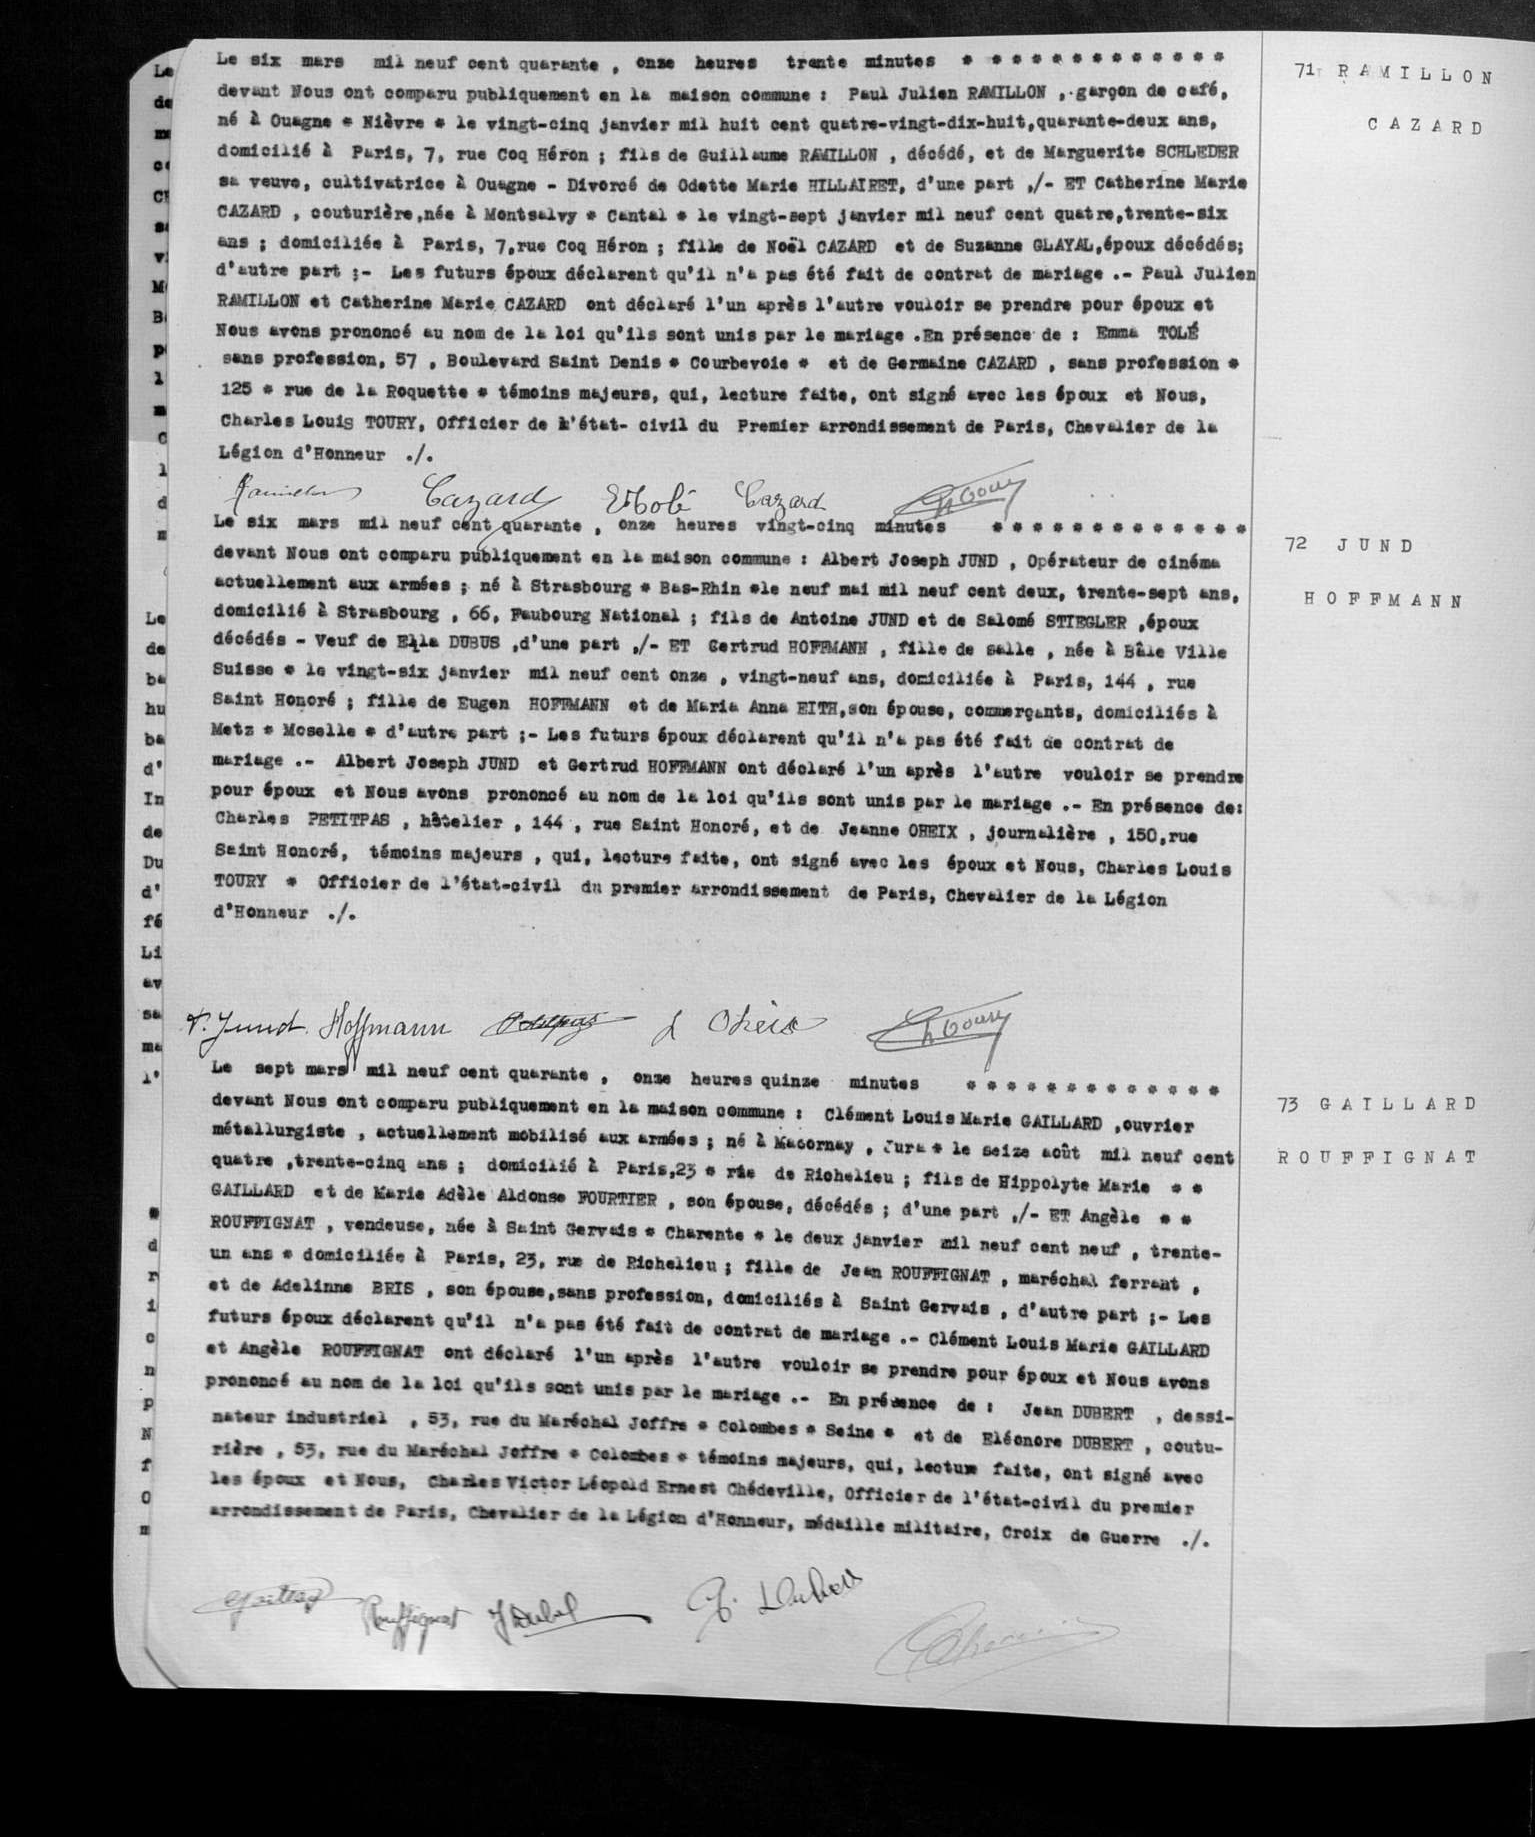Le six mars mil neuf cent quarante, onze heures trente minutes ****
devant Nous ont comparu publiquement en la maison commune: Paul Julien RAMILLON, garçon de café,
né à Ouagne * Nièvre * le vingt-cinq janvier mil huit cent quatre-vingt-dix-huit, quarante-deux ans,
domicilié à Paris, 7, rue Coq Héron; fils de Guillaume RAMILLON, décédé, et de Marguerite SCHLEDER
sa veuve, cultivatrice Ouagne-Divorcé de Odette Marie HILLAIRET, d'une part ,/-Et Catherine Marie
CAZARD, couturière, née à Montsalvy * Cantal * le vingt-sept janvier mil neuf cent quatre, trente-six
ans; domiciliée à Paris, 7, rue Coq Héron; fille de Noël CAZARD et de Suzanne GLAYAL, époux décédés;
d'autre part ;-Les futurs époux déclarent qu'il n'a pas été fait de contrat de mariage .-Paul Julien
RAMILLON et Catherine Marie CAZARD ont déclaré l'un après l'autre vouloir e prendre pour époux et
Nous avons prononcé au nom de la loi qu'ils sont unis par le mariage. En présence de: Emma TOLE
sans profession, 57, Boulevard Saint Denis * Courbevoie * et de Germaine CAZARD, sans profession *
125 * rue de la Roquette * témoins majeurs, qui, lecture faite, ont signé avec les époux et Nous,
Charles Louis TOURY, Officier de l'état-civil du Premier arrondissement de Paris, Chevalier de la
Légion d'Honneur ./.
Le six mars mil neuf cent quarante, onze heures vingt-cinq minutes ****
devant Nous ont comparu publiquement en la maison commune: Albert Joseph JUND, Opérateur de cinéma
actuellement aux armées; né à Strasbourg * Bas-Rhin * le neuf mai mil neuf cent deux, trente-sept ans,
domicilié à Strasbourg, 66, Faubourg National; fils de Antoine JUND et de Salomé STIELGLER, époux
décédés-Veuf de Ella DUBUS, d'une part ,/-ET Gertrud HOFFMAN, fille de salle, née à Bâle Ville
Suisse * le vingt-six janvier mil neuf cent onze, vingt-neuf ans, domiciliée à Paris, 144, rue
Saint Honoré; fille de Eugen HOFFMAN et de Maria Anna EITH, son épouse, commerçants, domiciliés à
Metz * Moselle * d'autre part ;-Les futurs époux déclarent qu'il n'a pas été fait de contrat de
mariage .-Albert Joseph JUND et Gertrud HOFFMAN ont déclaré l'un après l'autre vouloir se prendre
pour époux et Nous avons prononcé au nom de la loi qu'ils sont unis par le mariage .-En présence de:
Charles PETITPAS, hôtelier, 144, rue saint Honoré, et de Jeanne OHEIX, journalière, 150, rue
Saint Honoré, témoins majeurs, qui, lecturs faites, ont signé avec les époux et Nous, Charles Louis
TOURY * Officier de l'état-civil du premier arrondissement de Paris, Chevalier de la Légion
d'Honneur ./.
Le sept mars mil neuf cent quarante, onze heures quinze minutes ****
devant Nous ont comparu publiquement en la maison commune: Clément Louis Marie GAILLARD, ouvrier
métallurgiste, actuellement mobilisé aux armées; né à Macornay, Jura * le seize août mil neuf cent
quatre, trente-cinq ans; domicilié à Paris, 23 * ras de Richelieu; fils de Hippolyte Marie **
GAILLARD et de Marie Adèle Aldonse FOURTIER, son épouse, décédés; d'une part ,/-ET Angèle **
ROUFFIGNAT, vendeuse, née à Saint Gervais * Charente * le deux janvier mil neuf cent neuf, trente-
un ans * domiciliée à Paris, 23, rue de Richelieu; fille de Jean ROUFFIGNAT, maréchal ferrant,
et de Adelinne BRIS, son épouse, sans profession, domiciliés à Saint Gervais, d'autre part:-Les
futurs époux déclarent qu'il n'a pas été fait de contrat de mariage .-Clément Louis Marie GAILLARD
et Angèle ROUFFIGNAT ont déclaré l'un après l'autre vouloir se prendre pour époux et Nous avons
prononcé au nom de la loi qu'ils sont unis par le mariage .-En présence de: Jean DUBERT, dessi-
nateur industriel, 53, rue du Maréchal Joffre * Colombes * Seine * et de Eléonore DUBERT, coutu-
rière, 53, rue du Maréchal Joffre * Colombes * témoins majeurs, qui, lecture faite, ont signé avec
les époux et Nous, Charles Victor Léopold Ernest Chédeville, Officier de l'état-civil du premier
arrondissement de Paris, Chevalier de la Légion d'Honneur, médaille militaire, Croix de Guerre ./.
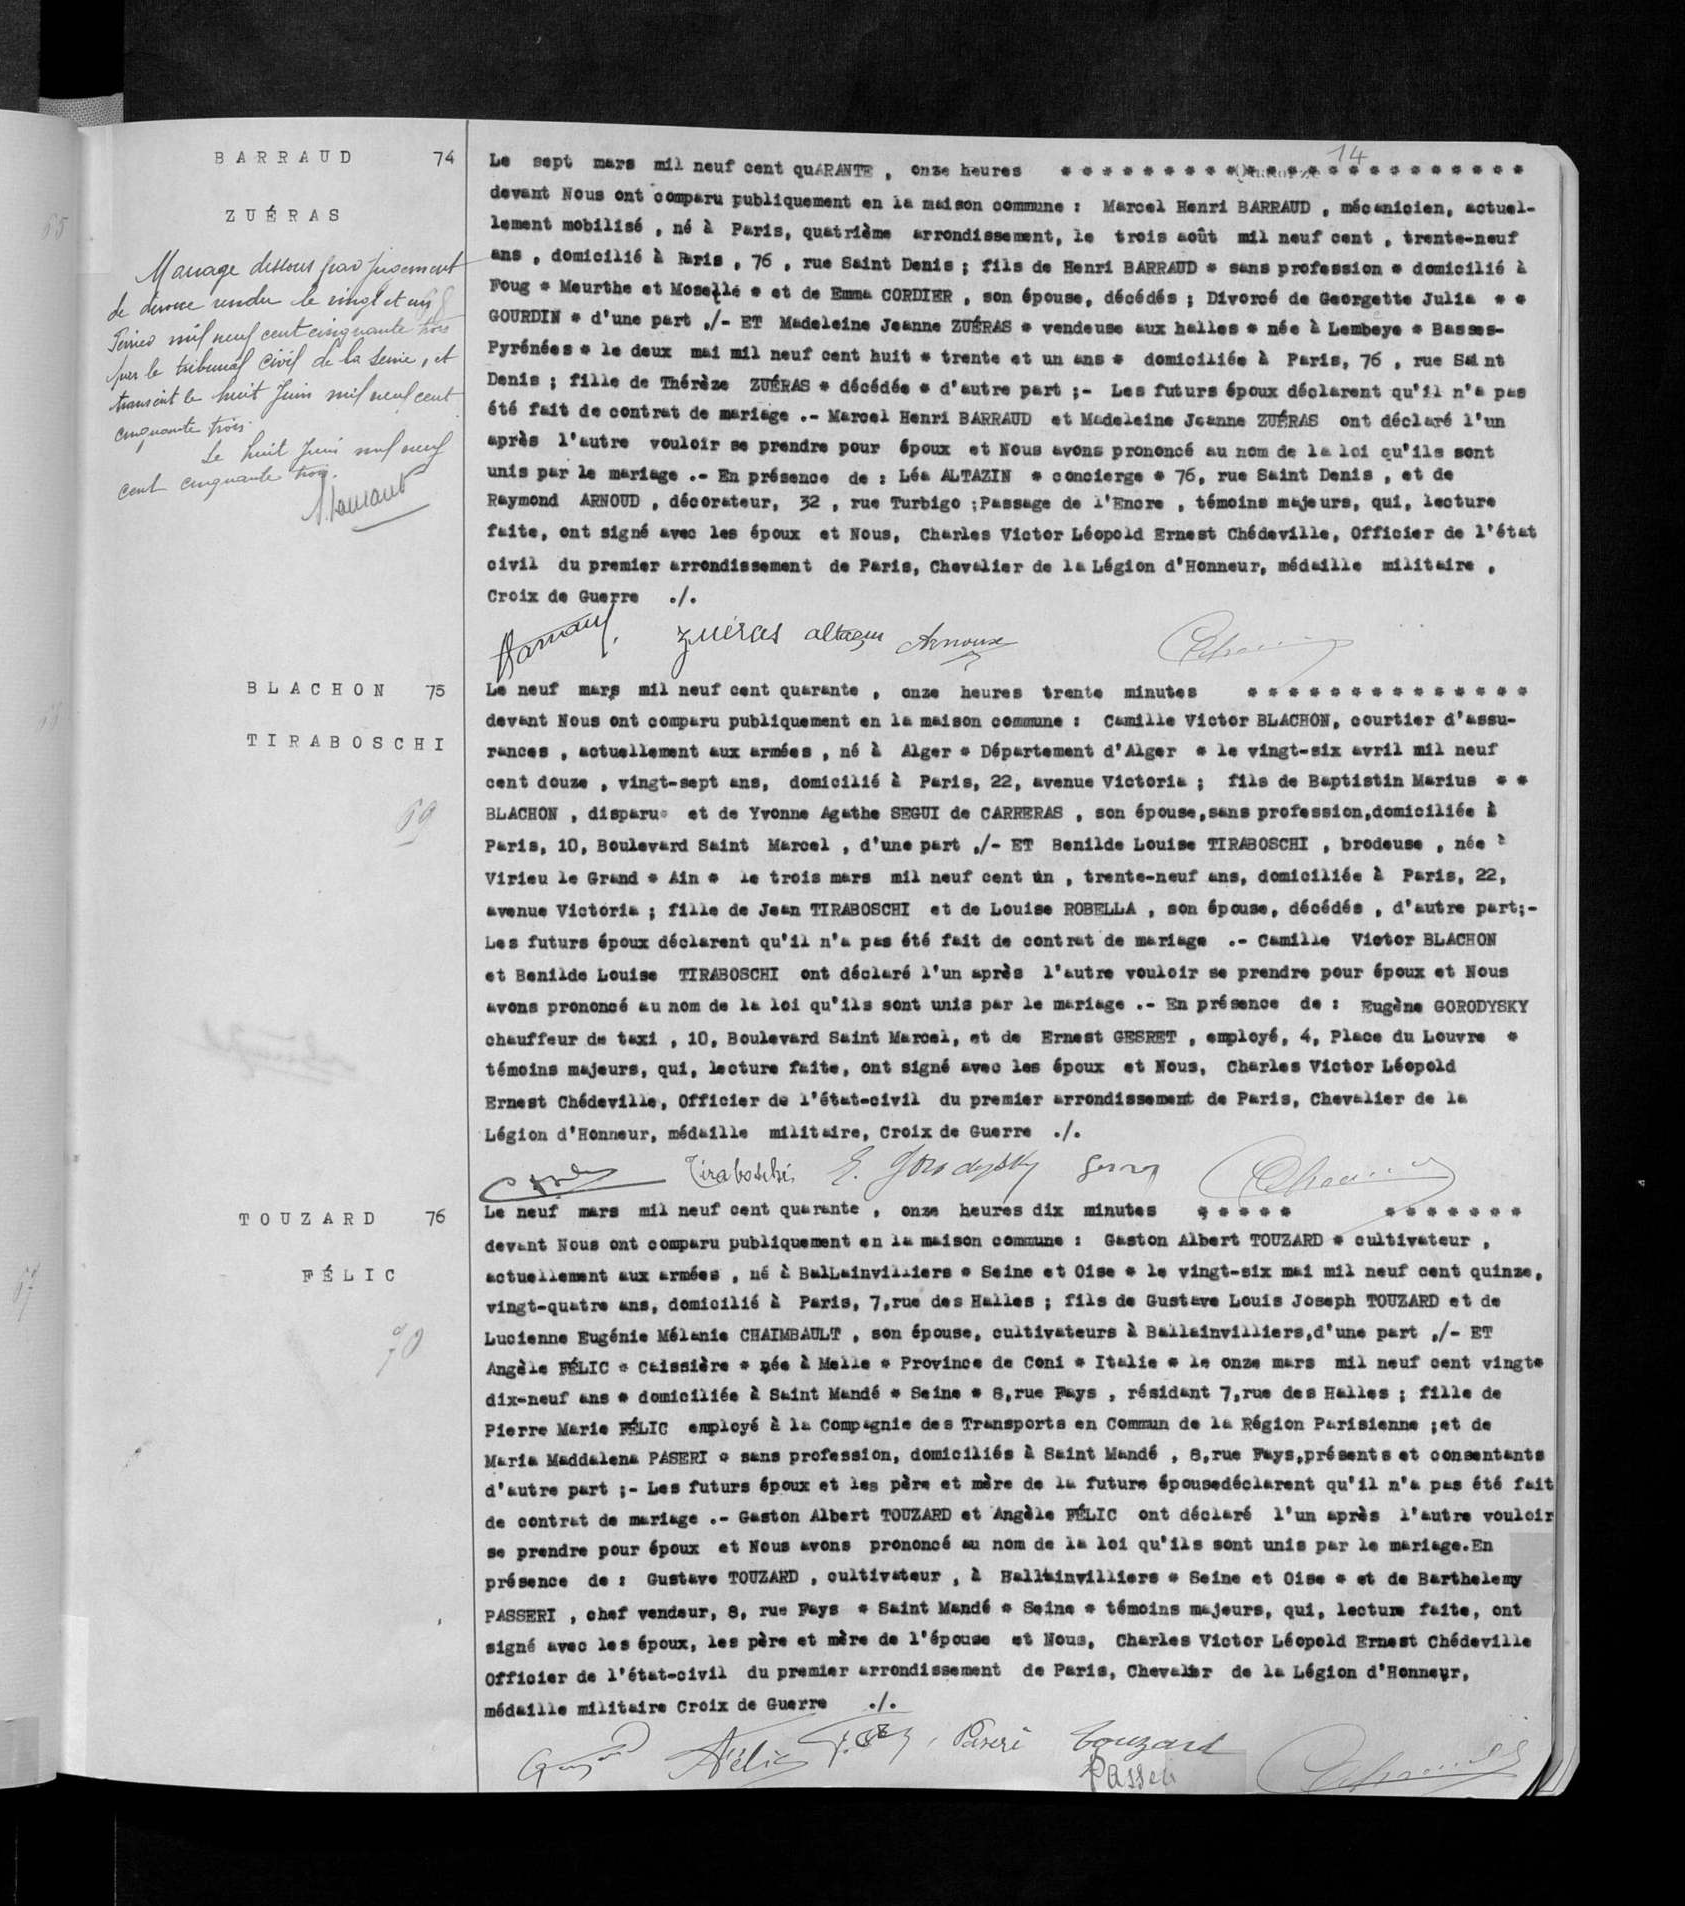Le sept mars mil neuf cent quarante, onze heures ****
devant Nous ont comparu publiquement en la maison commune: Marcel Henri BARRAUD, mécanicien, actuel-
lement mobilisé, né à Paris, quatrième arrondissement, le trois août mil neuf cent, trente-neuf
ans, domicilié à Paris, 76, rue Saint Denis; fils de Henri BARRAUD * sans profession * domicilié à
Foug * Meurthe et Moselle * et de Emma CORDIER, son épouse, décédés; Divorcé de Georgette Julia **
GOURDIN * d'une part ,/-ET Madeleine Jeanne ZUERAS * vendeuse aux halles * née à Lembeye * Basses-
Pyrénées * le deux mai mil neuf cent huit * trente et un ans * domiciliée à Paris, 76, rue Saint
Denis, fille de Thérèse ZUERAS * décédée * d'autre part ;-Les futurs époux déclarent qu'il n'a pas
été fait de contrat de mariage .-Marcel Henri BARRAUD et Madeleine Joanne ZUERAS ont déclaré l'un
après l'autre vouloir se prendre pour époux et Nous avons prononcé au nom de la loi qu'ils sont
unis par le mariage .-En présence de: Léa ALTAZIN * concierge * 76, rue Saint Denis, et de
Raymon ARNOUD, décorateur, 32, rue Turbigo; Passage de l'Eore, témoins majeurs, qui, lecture
faite ont signé avec les époux et Nous, Charles Victor Léopold Ernest Chédeville, Officier de l'état
civil du premier arrondissement de Paris, Chevalier de la Légion d'Honneur, médaille militaire,
Croix de Guerre ./.
Le neuf mars mil neuf cent quarante, onze heures trente minutes ****
devant Nous ont comparu publiquement en la maison commune: Camille Victor BLACHON, courtier d'assu-
rances, actuellement aux armées, né à Alger * Département d'Alger * le vingt-six avril mil neuf
cent douze, vingt-sept ans, domicilié à Paris, 22, avenue Victoria; fils de Baptistin Marius **
BLACHON, disparue et de Yvonne Agathe SEGUI de CARRERAS, son épouse, sans profession, domiciliée à
Paris, 10, Boulevard Saint Marcel, d'une part ,/-ET Benilde Louise TIRABOSCHI, brodeuse, née à
Virieu le Grand * Ain * le trois mars mil neuf cent un, trente-neuf ans, domiciliée à paris, 22,
avenue Victoria; fille de Jean TIRABOSCHI et de Louise ROBELLA, son épouse, décédés, d'autre part ;-
Les futurs époux déclarent qu'il n'a pas été fait de contrat de mariage .-Camille Victor BLACHON
et Benilde Louise TIRABOSCHI ont déclaré l'un après l'autre vouloir se prendre pour époux et Nous
avons prononcé au nom de la loi qu'ils sont unis par le mariage .-En présence de Eugène GORDYSKY
chauffeur de taxi, 10, Boulevard Saint Marcel, et de Ernest GESRET, employé, 4, Place du Louvre *
témoins majeurs, qui, lecture faite, ont signé avec les époux et Nous, Charles Victor Léopold
Ernest Chédeville, Officier de l'état-civil du premier arrondissement de Paris, Chevalier de la
Légion d'Honneur, médaille militaire, Croix de Guerre ./.
Le neuf mars mil neuf cent quarante, onze heures dix minutes ****
devant Nous ont comparu publiquement en la maison commune: Gaston Albert TOUZARD * cultivateur,
actuellement aux armées, né à BalLainvilliers * Seine et Oise * le vingt-six mai mil neuf cent quinze,
vingt-quatre ans, domicilié à Paris, 7, rue des Halles; fils de Gustave Louis Joseph TOUZARD et de
Lucienne Eugénie Mélanie CHAIMBAULT, son épouse, cultivateurs à Ballaivilliers, d'une part ,/-ET
Angèle FELIC * Caissière * née à Melle * Province de Coni * Italie * le onze mars mil neuf cent vingt*
dix-neuf ans * domicilié à saint Mandé * Seine * 8, rue Fays, résidant 7, rue des Halles; fille de
Pierre Marie FELIC employé à la Compagnie des Transports en Commun de la Région Parisienne; et de
Maria Maddalena PASERI * sans profession, domiciliés à Saint Mandé, 8, rue Fays, présent et consentants
d'autre part ;-Les futurs époux et les père et mère de la future épouse déclarent qu'il n'a pas été fait
de contrat de mariage .-Gaston Albert TOUZARD et Angèle FELIC ont déclaré l'un après l'autre vouloir
se prendre pour époux et Nous avons prononcé au nom de la loi qu'ils sont unis par le mariage. En
présence de: Gustave TOUZARD, cultivateur, à Hallainvilliers * Seine et Oise * et de Barthelemy
PASSERI, chef vendeur, 8, rue Fays * Saint Mandé * Seine * témoins majeurs, qui, lecture faite, ont
signé avec les époux, les père et mère de l'épouse et Nous, Charles Victor Léopold Ernest Chédeville
Officier de l'état-civil du premier arrondissement de Paris, Chevalier de la Légion d'Honneur,
médaille militaire Croix de Guerre ./.